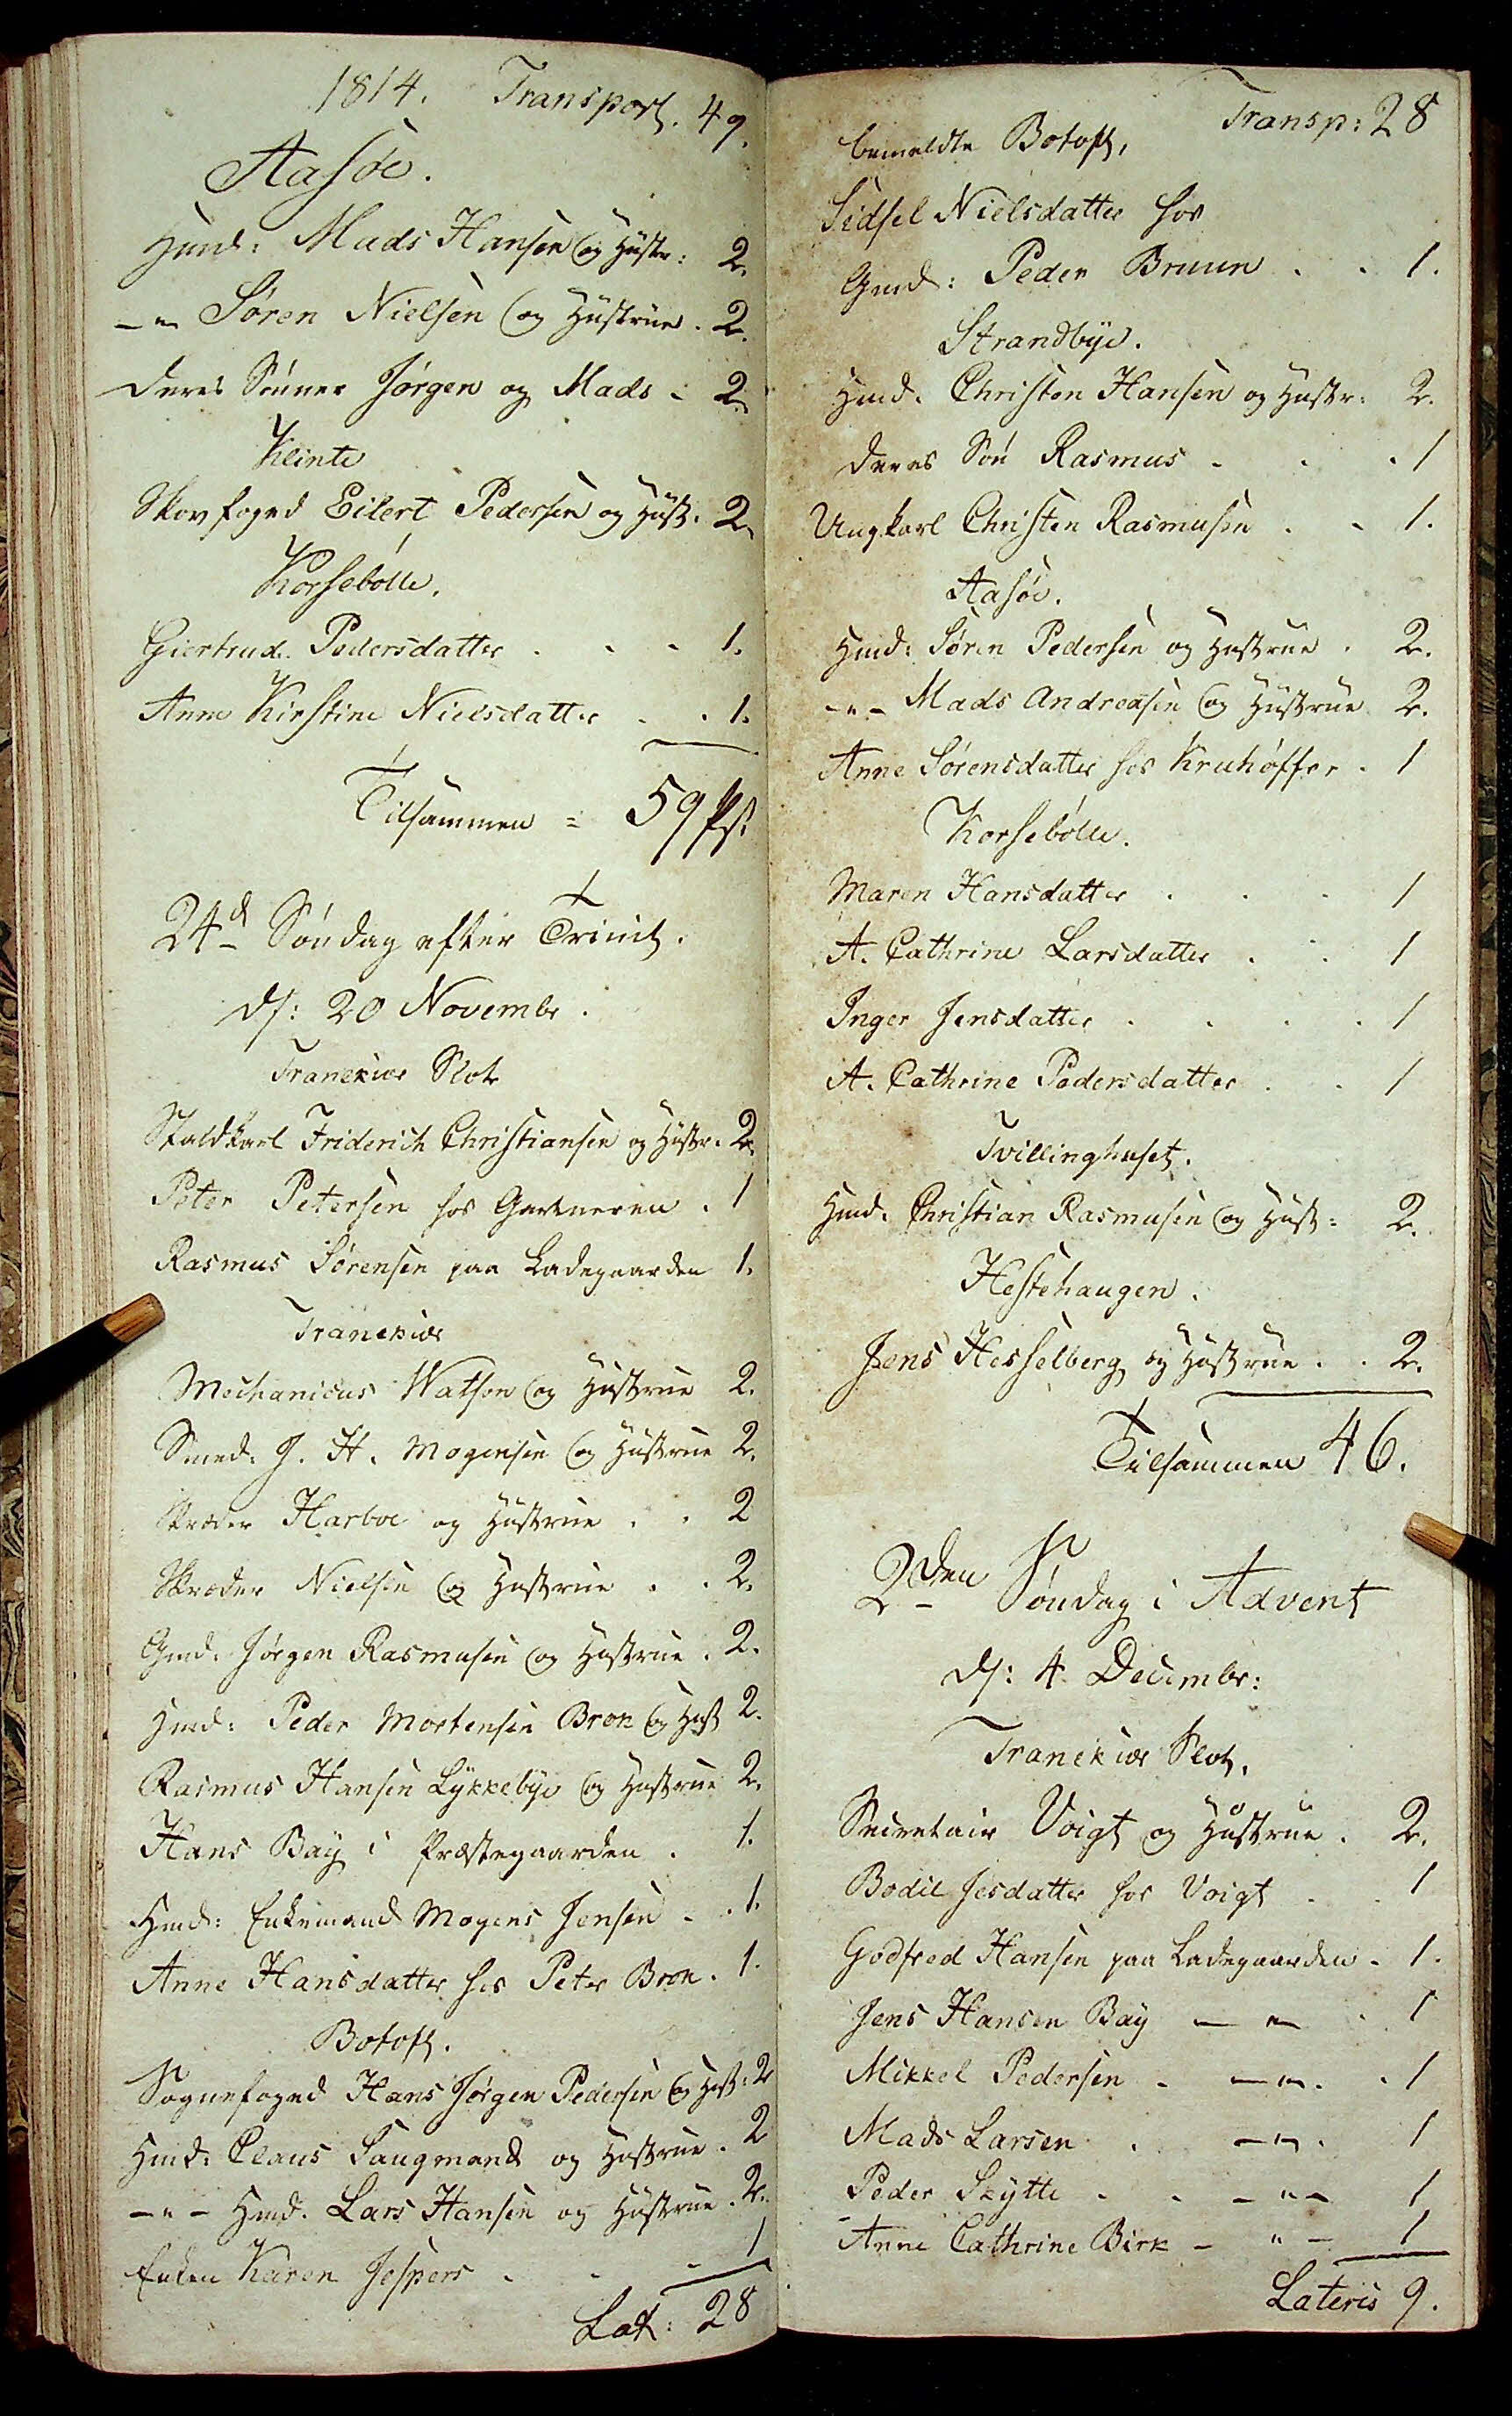1814.
Transport . 49.
Aasøe.
Hmd. Mads Hansens Hustr : 2.
-"- Søren Nielsen og Hustrue . 2.
deres Sønner Jørgen og Mads - 2
Kiente
Skovfoged Eilert Pedersen og Hust . 2.
Korsebølle.
Giertrud Pedersdatter . . . 1.
Anne Kirstine Nielsdatter . . 1.
Tilsammen = 59 Ps.
24de Søndag efter Trinit.
d: 20 Novembr.
Tranekiæe Slot
Staldkarl Friderich Christiansen og Hustr . 2.
Peter Petersen hos Gartneren . 1
Rasmus Sørensen paa Ladegaarden 1.
Tranekiær
Mechanicus Watson og Hustrue 2.
Smed. J. H. Mogensen og Hustrue 2.
Peder Harboe og Hustrue . . 2
Skræder Nielsen og Hustrue . . 2.
Gmd: Jørgen Rasmusen og Hustrue . 2.
Hmd: Peder Mortensen Brok og Hust 2.
Rasmus Hansen Lykkebye og Hustrue 2.
Hans Bay i Præstegaarden . 1.
Hmd: Enkemand Mogens Jensen . .1.
Anne Hansdatter hos Peter Brok . 1.
Botoft.
Sognefoged Hans Jørgen Pedersen og Hust . 2
Hmd: Claus Saugmand og Hustrue . 2
-"- Hmd. Lars Hansen og Hustrue . 2.
Enken Karen Jespers . . . 1
Lat: 28
Transp: 28
bemeldte Botoft.
Sidsel Nielsdatter hos
Gmd: Peder Bruun . . 1.
Strandbye.
Hmd. Christen Hansen og Hustr: 2.
deres Søn Rasmus . . . 1
Ungkarl Christen Rasmusen . . 1.
Aasøe.
Hmd, Søren Pedersen og Hustrue . 2.
-"- Mads Andreasen og Hustrue 2.
Anne Sørensdatter hos Kruhøffer . 1
Korsebølle.
Maren Hansdatter . . . 1
A. Cathrine Larsdatter .
Inger Jensdatter . . 1
A. Cathrine Pedersdatter . . 1
Tvillingset.
Hmd: Christian Rasmusen og Hust: 2.
Hestehaugen.
Jens Hisselberg og Hustrue . . 2.
Tilsammen 46.
2den Søndag i Advent
d: 4. Decembr:
Tranekiær Slot
Secretair Voigt og Hustrue . 2.
Bodil Jesdatter hos Voigt . .1
Godfred Hansen paa Ladegaarden . 1.
Jens Hansen Bay -"- . 1
Mikkel Pedersen . -"- . 1
Mads Larsen . -"- . 1
Peder Skytte . . -"- 1
Anne Cathrine Birk -"- 1
Lateris 9.
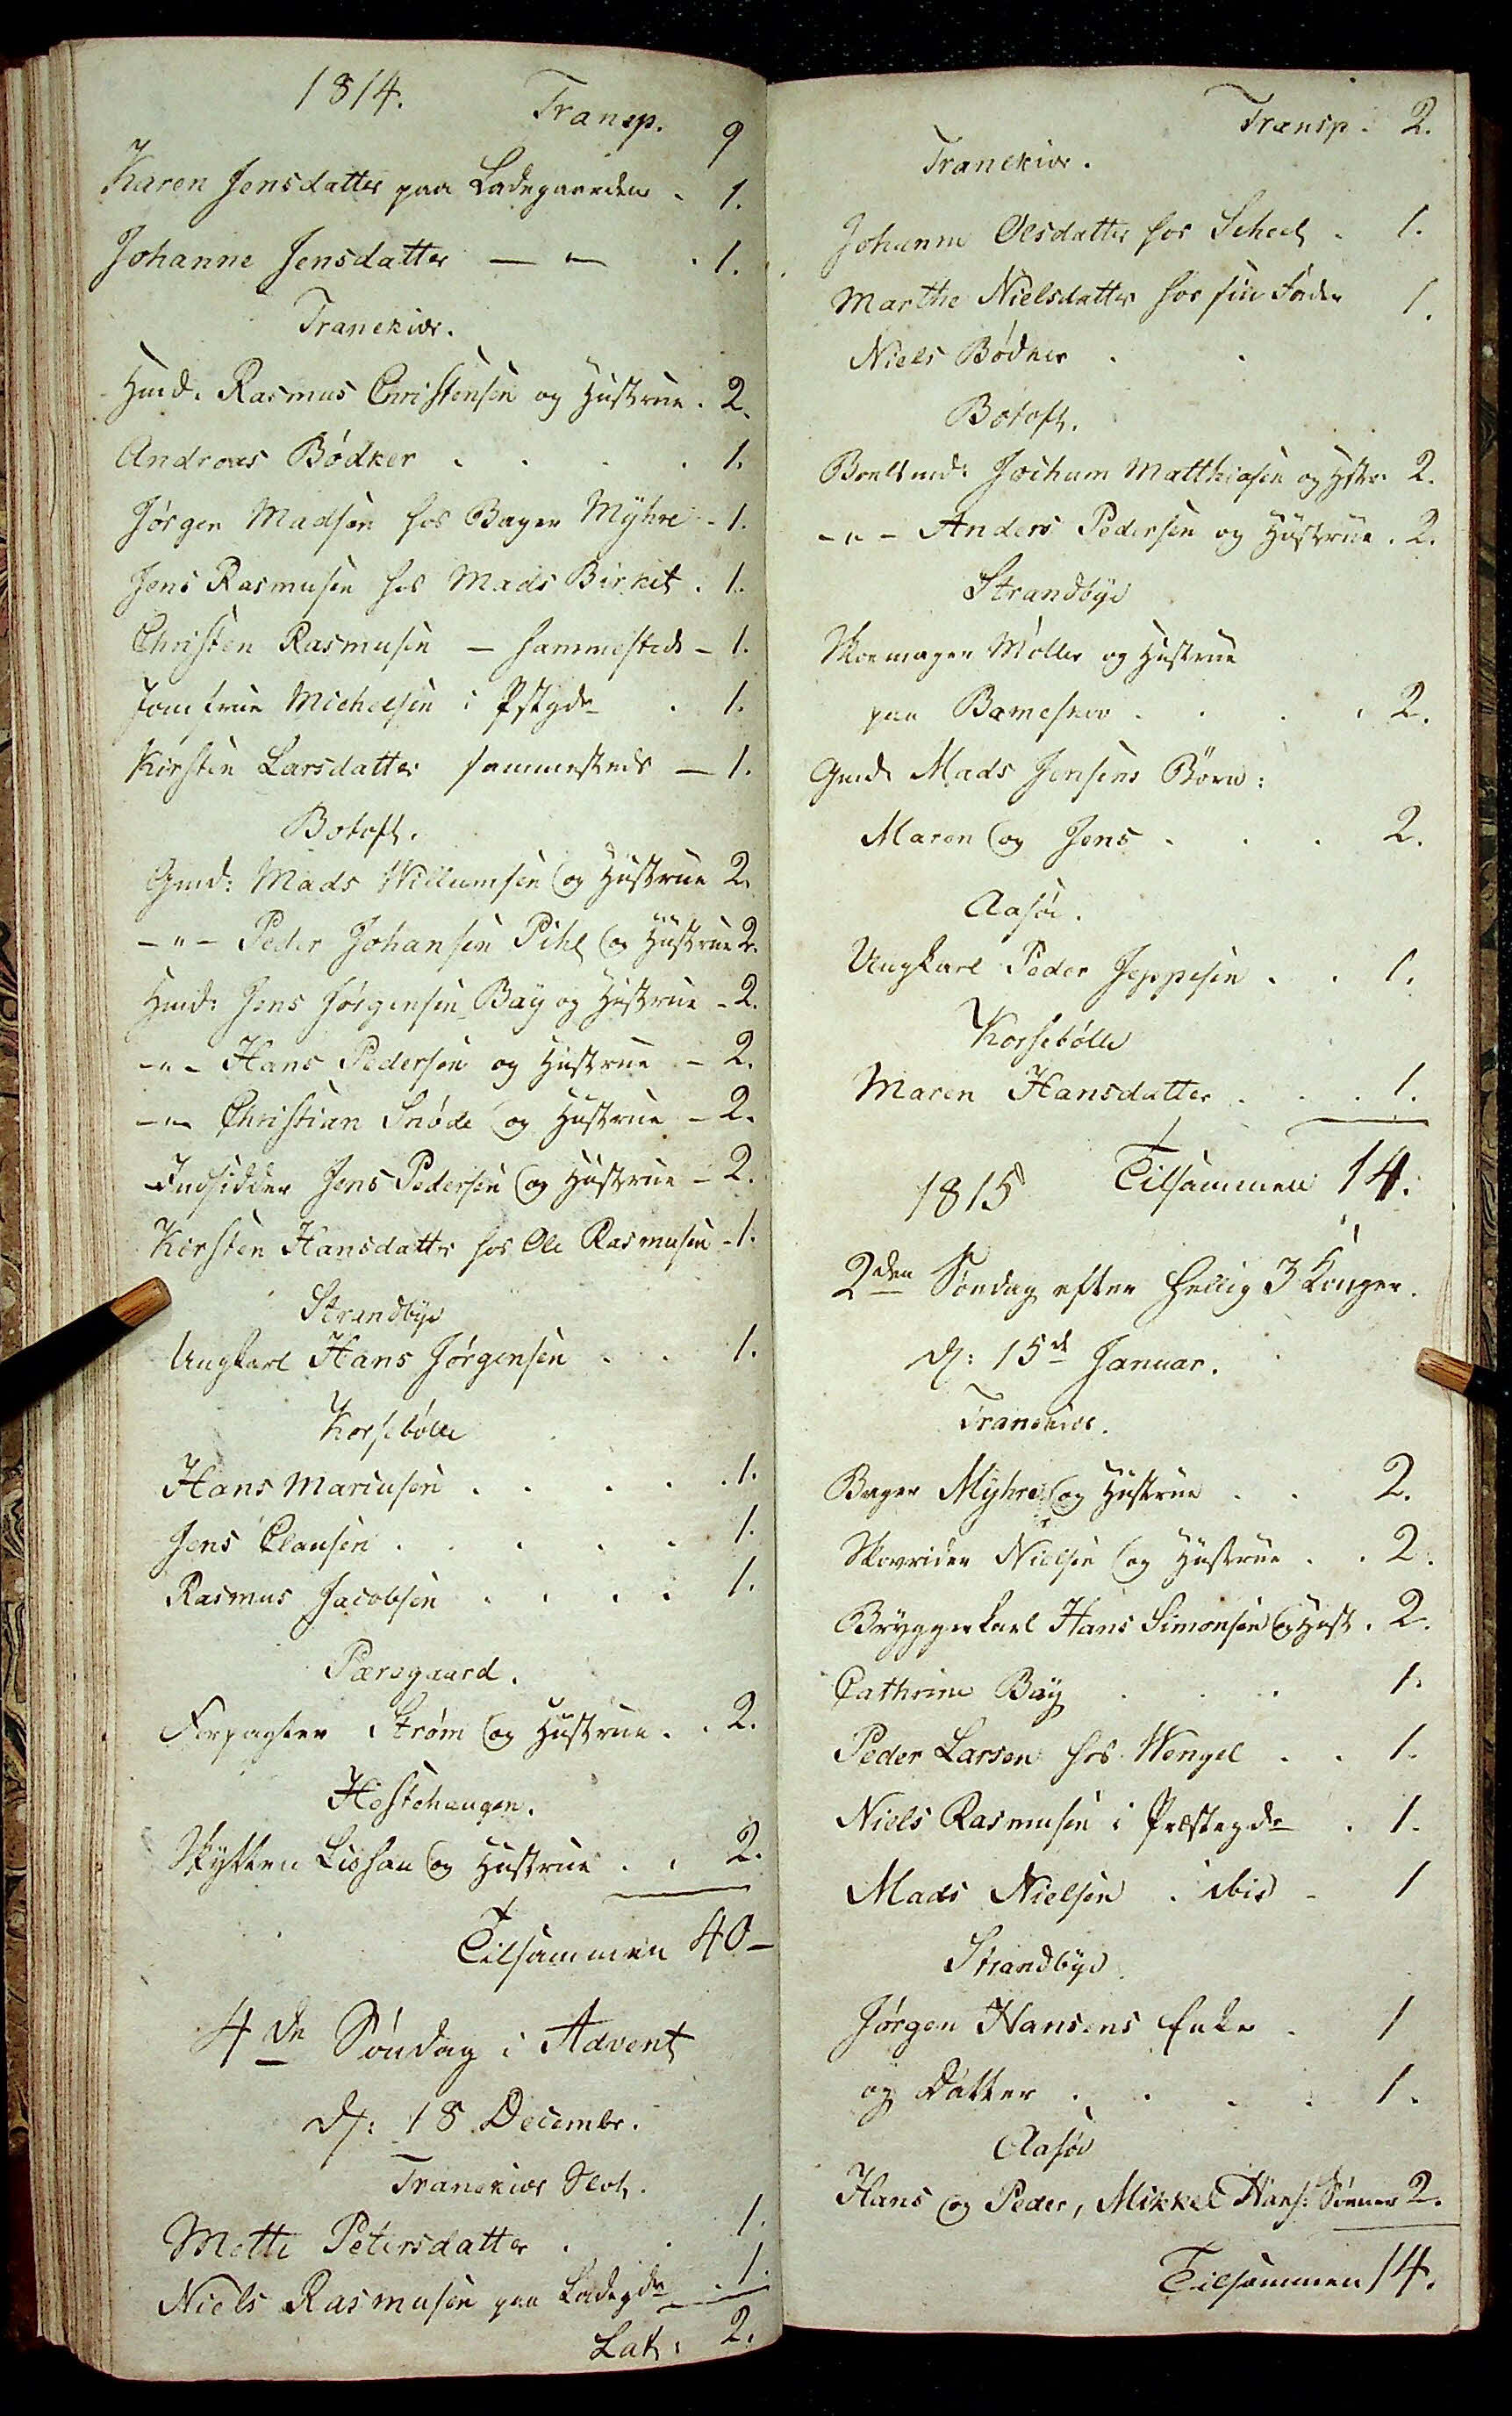1814.
Transp. 9
Karen Jensdatter paa Ladegaarden . 1.
Johanne Jensdatter -"- .1.
Tranekiær.
Hmd. Rasmus Christensen og Hustrue . 2.
Andreas Bødker . . . . 1.
Jørgen Madsen hos Bager Myhre . 1.
Jens Rasmusen hos Mads Birket . 1.
Christen Rasmusen - sammesteds - 1.
Jomfrue Michelsen i Pstgdr . 1.
Kirsten Larsdatter sammesteds - 1.
Botoft.
Gmd: Mads Willumsen og Hustrue 2.
-"- Peder Johansen Pihl og Hustrue 2.
Hmd: Jens Jørgensen Bay og Hustrue - 2.
-"- Hans Pedersen og Hustrue - 2.
-"- Christian Snøde og Hustrue - 2.
Indsidder Jens Pedersen og Hustrue - 2.
Kirsten Hansdatter hos Ole Rasmusen - 1.
Strandbye
Ungkarl Hans Jørgensen . .1.
Korsebølle
Hans Marcusen . . . . . 1.
Jens Clausen . . . . . 1.
Rasmus Jacobsen . . . . 1.
Pæregaard.
Forpagter Strøm og Hustrue . . 2.
Hestehaugen.
Skytten Lissau og Hustrue . . 2.
Tilsammen 40-
4de Søndag i Advent
d: 18 Decembr.
Tranekiær Slot
Mette Pedersdatter . . 1
Niels Rasmusen paa Ladegdr . 1
Lat: 2.
Transp . 2.
Tranekiær.
Johanne Olsdatter hos Scheel . 1.
Marthe Nielsdatter hos sin Fader 1.
Niels Bødker
Botoft.
Boelsmd: Jochum Matthiasen og Hstr: 2.
-"- Anders Pedersen og Hustrue . 2.
Strandbye
Skoemager Møller og Hustrue
paa Bameskov . . . . 2.
Gmd: Mads Jensens Børn:
Maren og Jens . . . 2.
Aasøe.
Ungkarl Peder Jeppesen . .1.
Korsebølle
Maren Hansdatter . . . 1. 
Tilsammen 14.
1815
2den Søndag efter Hellig 3 Konger.
d: 15de Januar.
Tranekiær.
Bager Myhre og Hustrue . . 2.
Skovrider Nielsen og Hustrue . . 2.
Bryggerkarl Hans Simonsen og Hust . 2.
Cathrine Bay . . . 1.
Peder Larsen hos Wengel . . 1.
Niels Rasmusen i Præstegdr . 1.
Mads Nielsen . ibid - 1
Strandbye
Jørgen Hansens Enke . 1
og Datter . . . . 1.
Aasøe
Hans og Peder, Mikkel Hans: Sønner: 2.
Tilsammen 14.